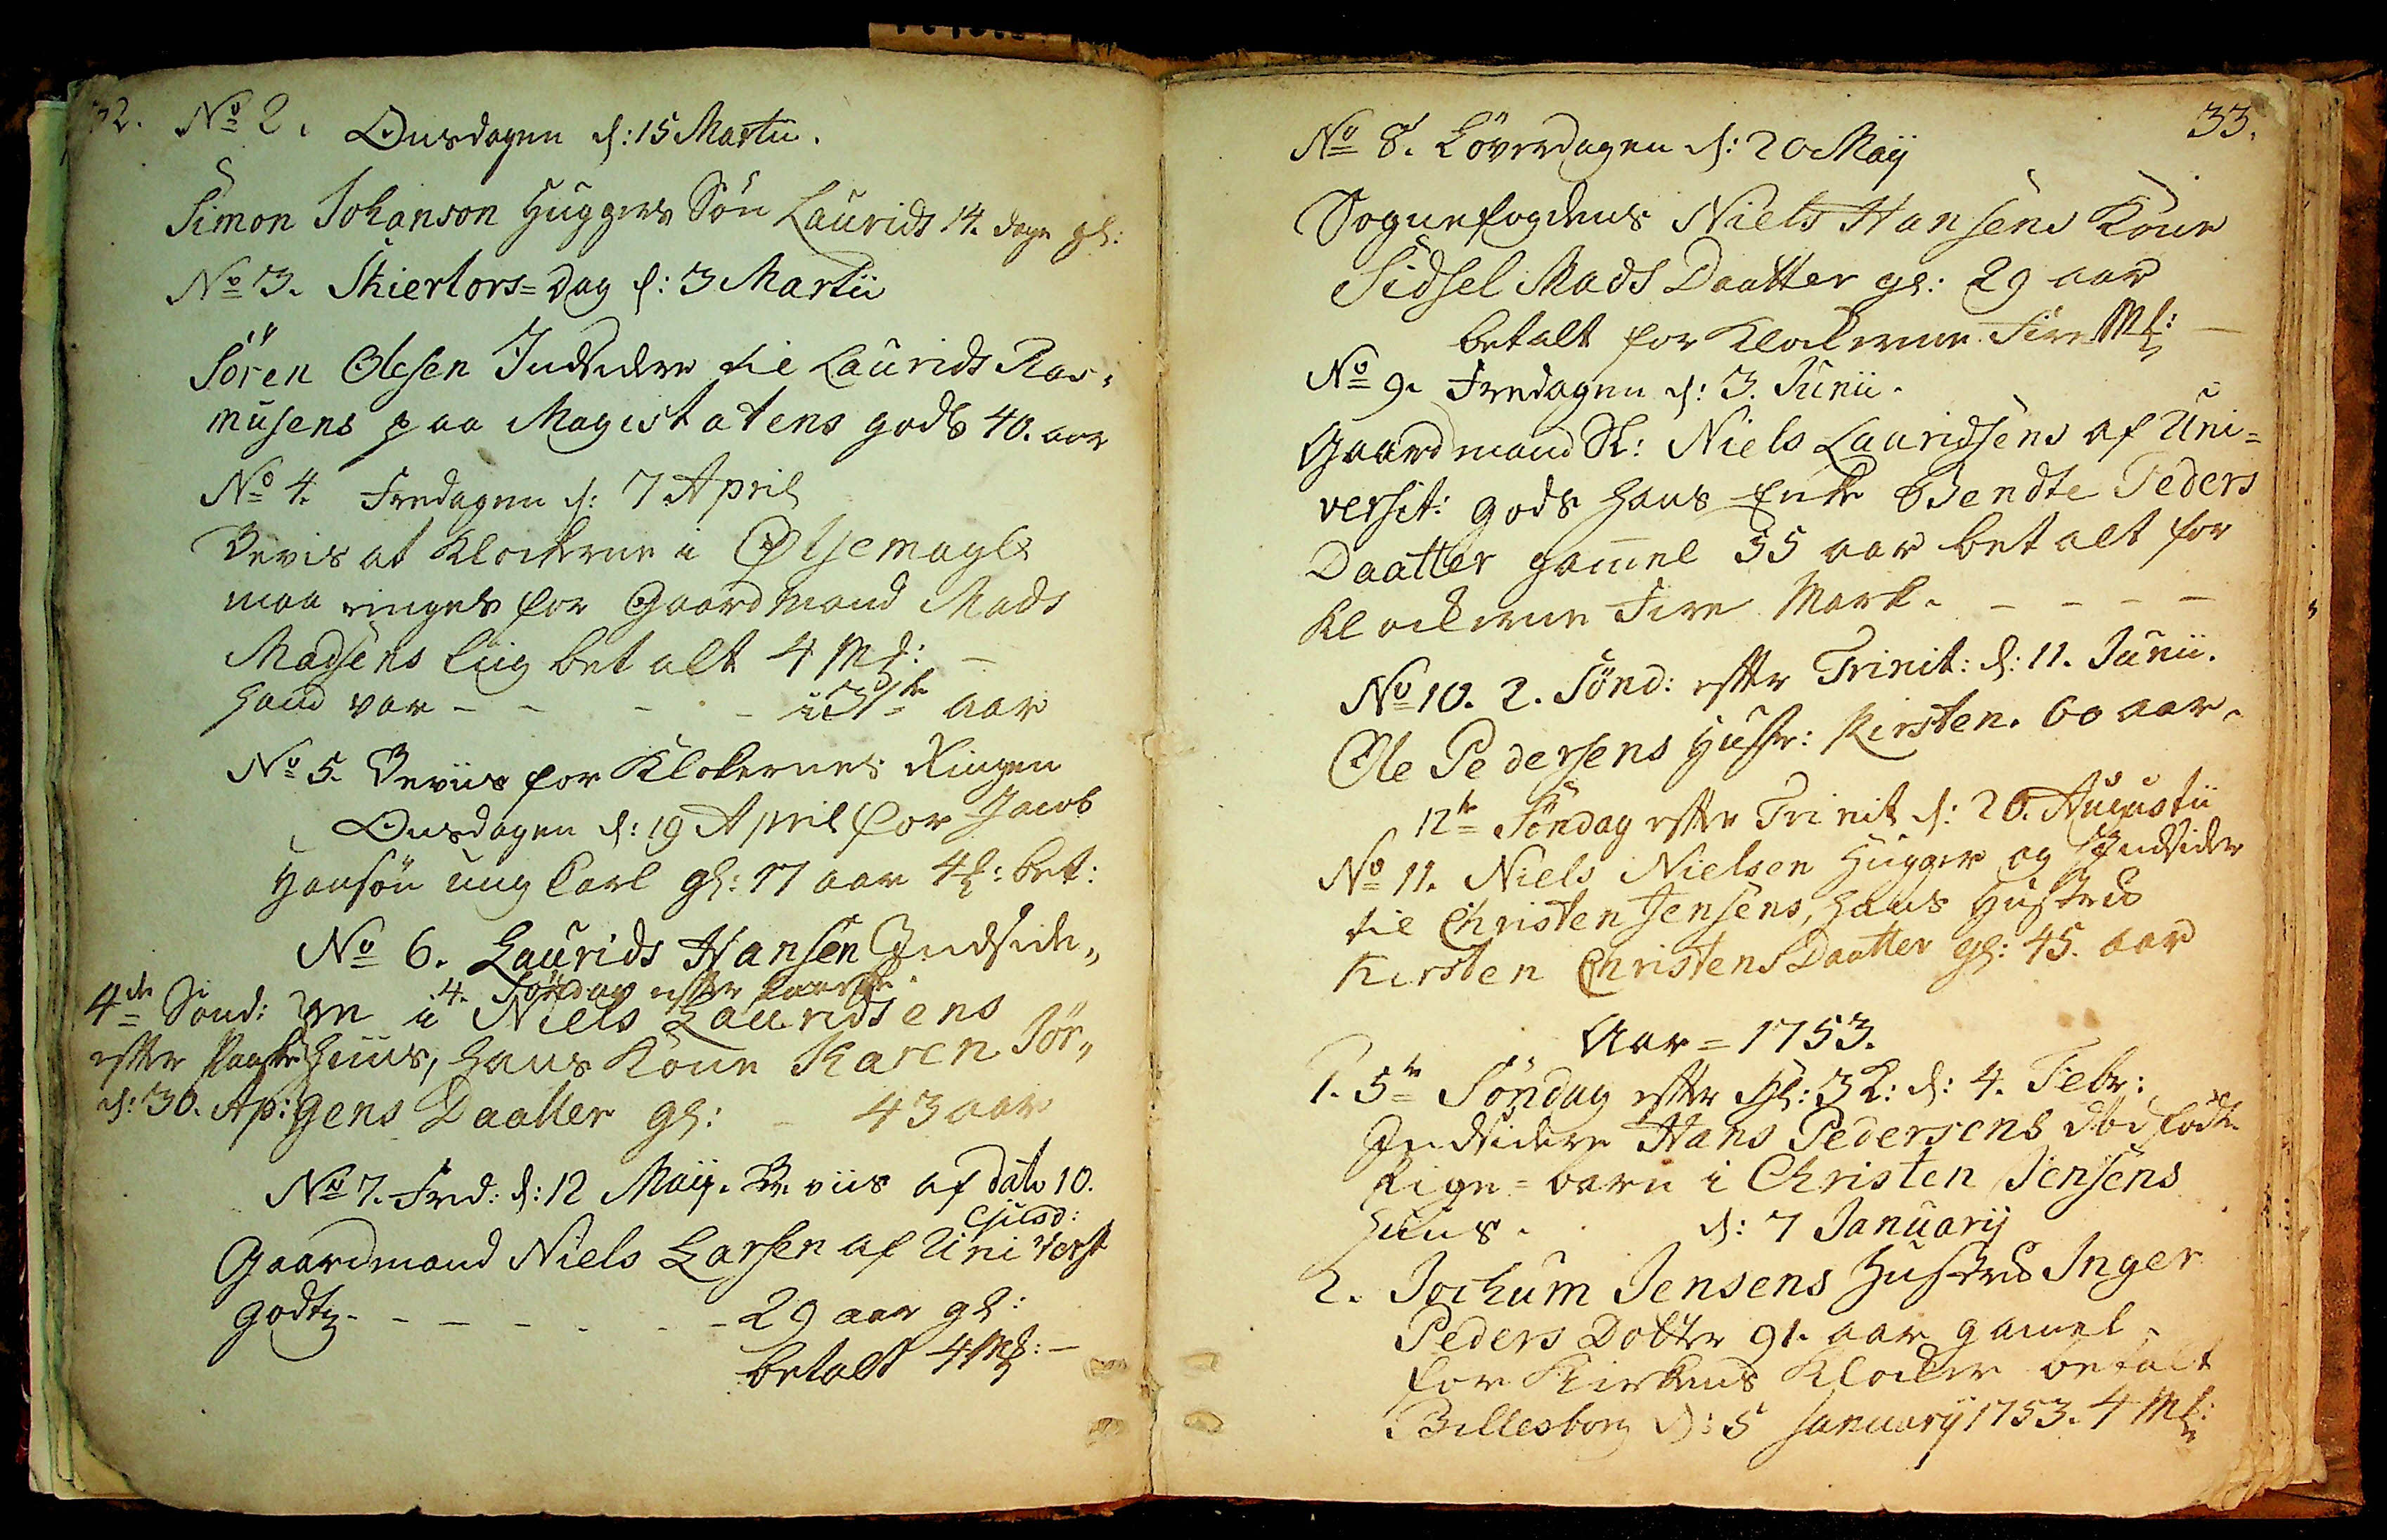32.
No 2. Onsdagen d: 15 Martii.
Simon Johanson Huggers Søn Laurids 14. dage gl:
No 3. Skiertors=Dag d: 3 Martii
Søren Olesen Indsider til Laurids Ras¬
musens paa Magistratens gods 40. aar
No 4. Fredagen d: 7 April
Bevis at Klockerne i Ølsemagle
maa ringes for Gaardmand Mads
Madsens Liig betalt 4 Mk:
Hand var
i 31te aar
No 5. Beviis for Klockernes Ringen
Onsdagen d: 19 April for Jacob
Hansøn Ung karl gl: 17 aar 4m: bet:
No 6. Laurids Hansen Jndsid¬
4. Søndag efter Paaske.
4de Sønd: er i Niels Lauridsens
efter Paaske Huus, hans Kone Karen Jør¬
d: 30.Ap: gens Daatter gl: - 43 aar
No 7. Fred: d: 12 Maij. Beviis af Dato 10.
Ejusd:
Gaardmand Niels Larsen af Universt
Godtz
29 aar gl:
betalt 4 Mk:
33.
No 8. Løverdagen d: 20 Maij
Sognefogedens Niels Hansens Kone
Sidsel Mads Daatter gl: 29 aar
betalt for Klockerne Fire Mk:
No 9. Fredagen d: 3. Junii.
Gaardmand Sl: Niels Lauridsens af Uni¬
versit: Gods hans Enke Bendte Peders
Daatter gammel 55 aar betalt for
Klockerne Fire Mark
No 10. 2. Sønd: efter Trinit: d: 11. Junii.
Ole Pedersens Hustr: Kirsten. 60 aar.
12te Søndag efter Trinit d: 20. Augustii
No 11. Niels Nielsen Hugger og Indsider
til Christen Jensens, hans Hustru
Kirsten Christens Daatter gl: 45. aar
Aar = 1753.
1. 5te Søndag efter Hl: 3 K: d: 4. Febr:
Indsider Hans Pedersens dødfødte
Pige=barn i Christen Jensens
Huus.
d: 7 Januarij
2. Jochum Jensens Hustru Inger
Peders Dotter 91. aar gammel.
for Kirkens Klocker betalt
Billesborg d: 5 Januarij 1753. 4 Mk:
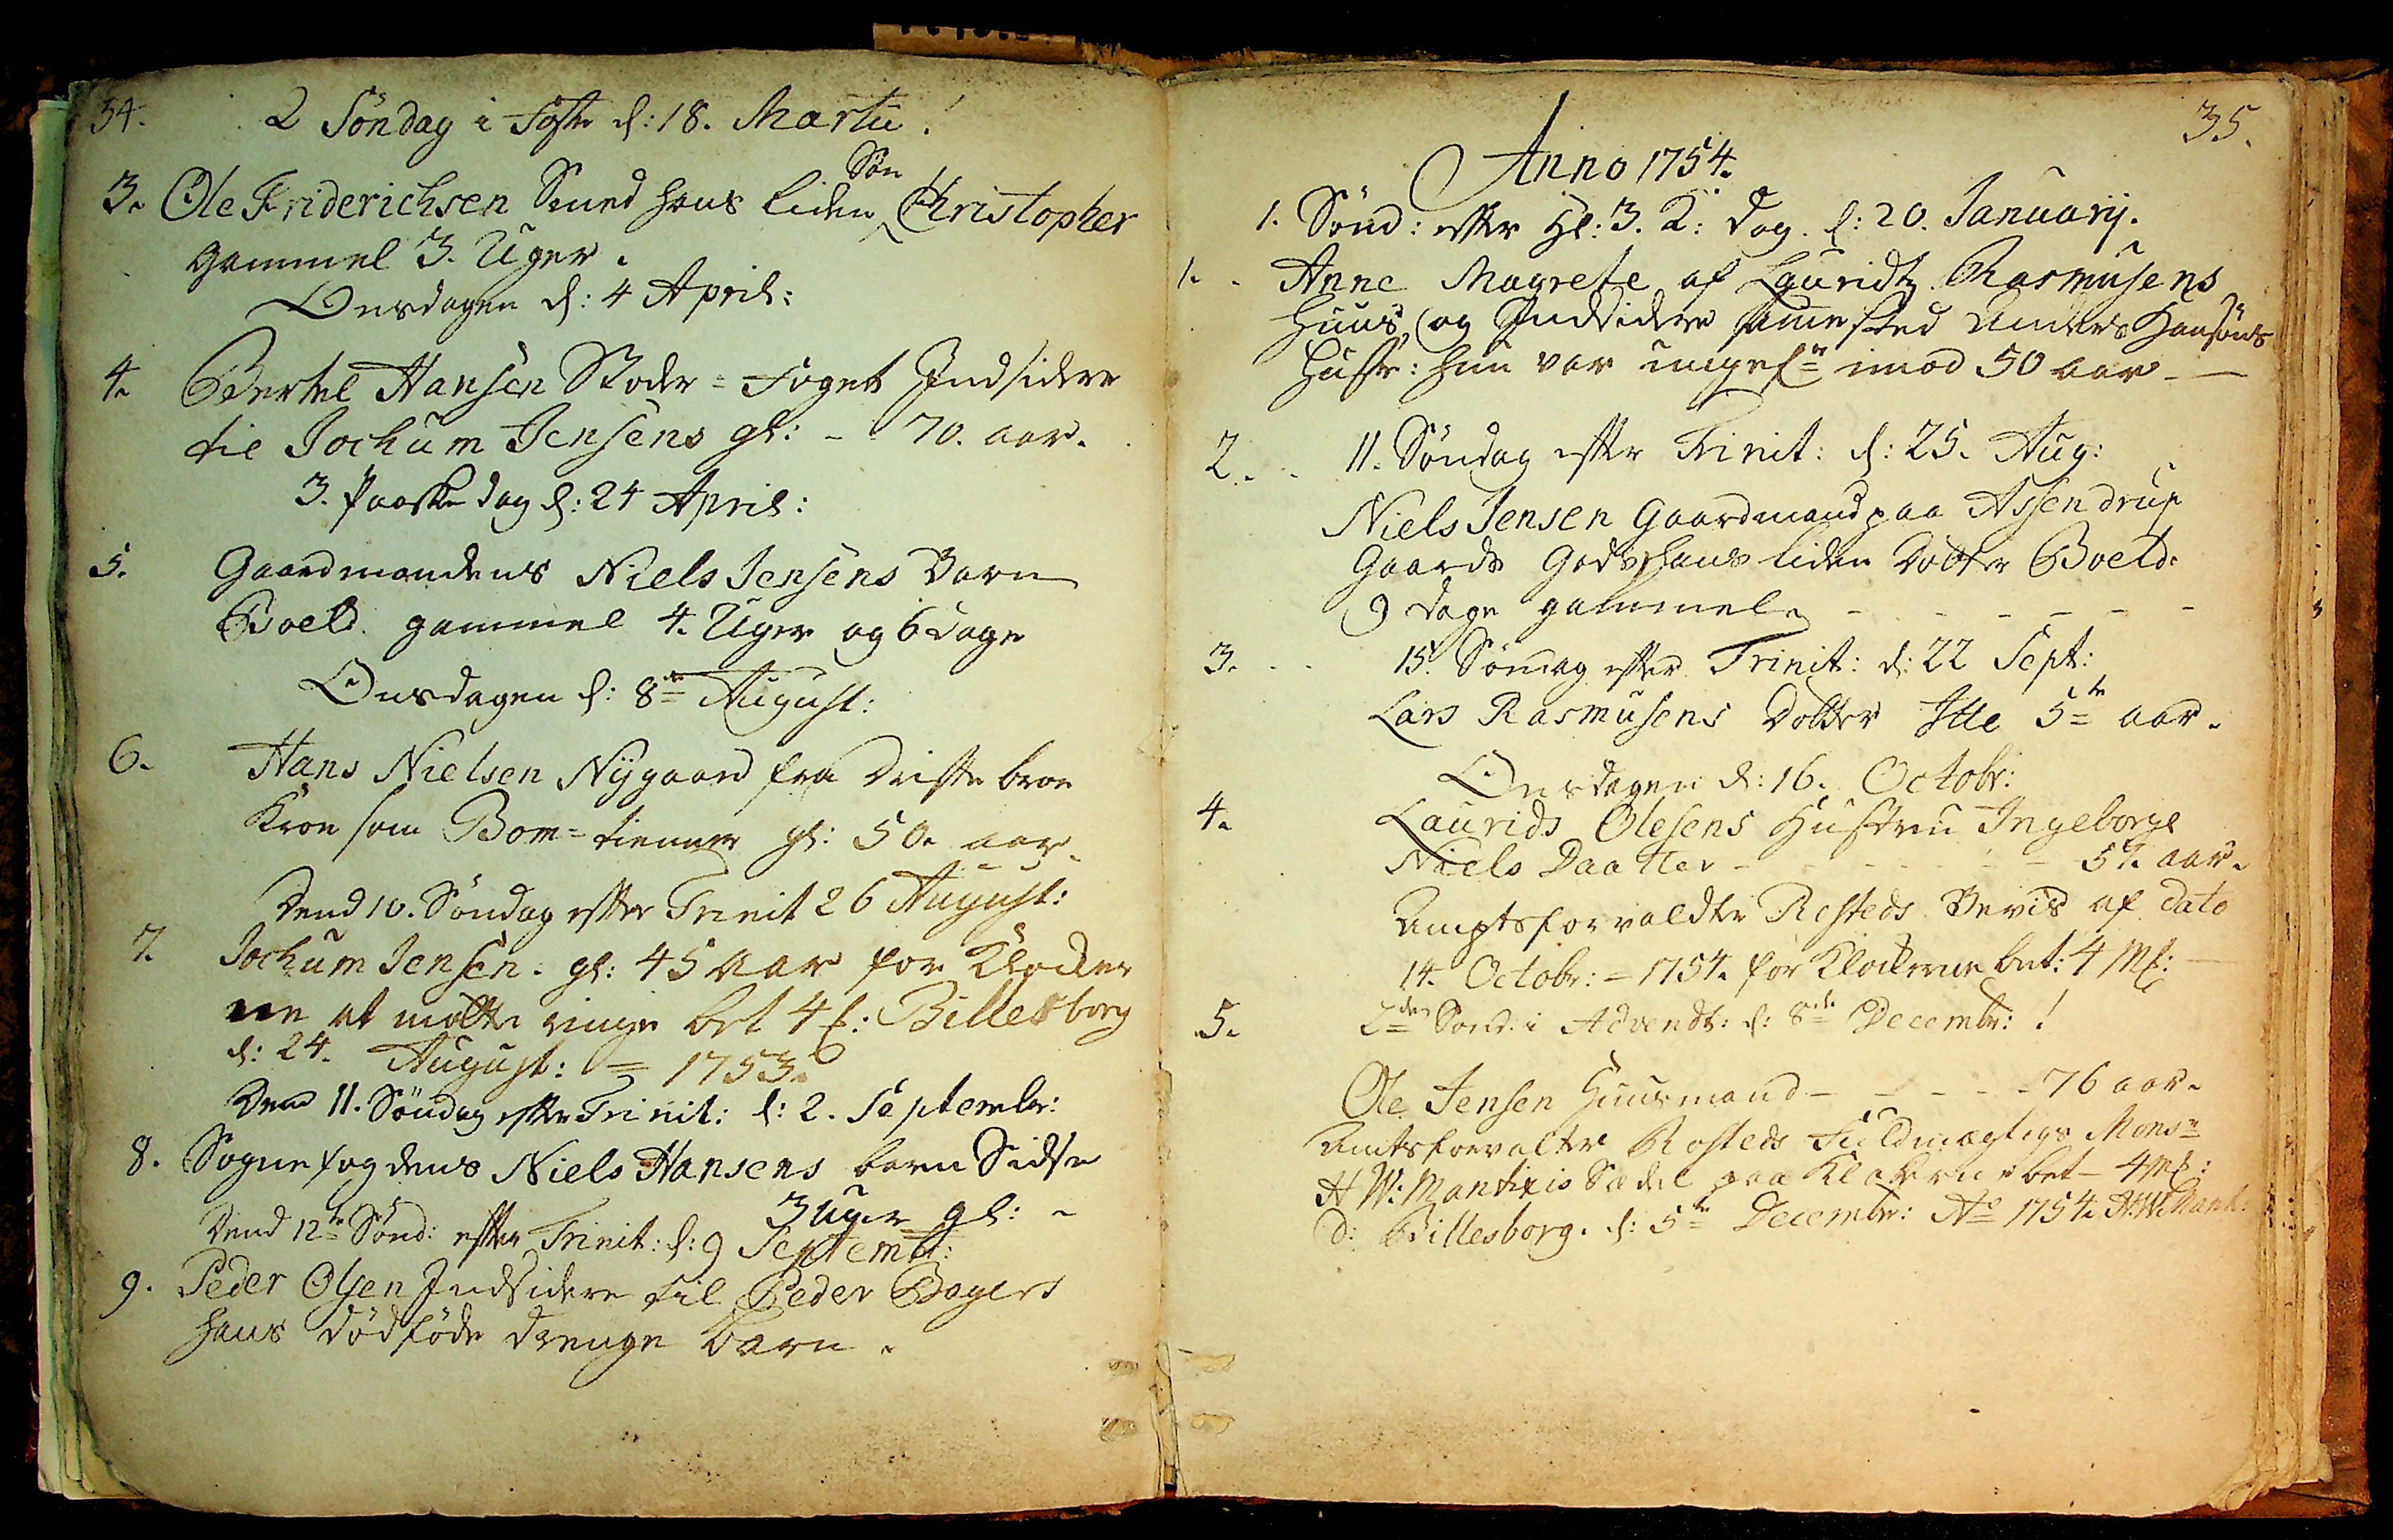34.
2 Søndag i Faste d: 18 Martii.
Søn
3. Ole Friderichsen Smed hans liden Christopher
Gammel 3. Uger.
Onsdagen d: 4 April:
4. Bertel Hansen Stoder=Foget Indsider
til Jochum Jensens gl: - 70. aar.
3. Paaskedag d. 24 April
5. Gaardmandens Niels Jensens Barn
Boeld. gammel 4. Uger og 6 Dage
Onsdagen d: 8de August:
6. Hans Nielsen Nygaard fra Driftebroe
Kroe som Bom=tiener gl: 50. aar.
Dend 10. Søndag efter Trinit 26 August:
7. Jochum Jensen. gl: 45 Aar for Klocker¬
ne at motte ringe bet 4 M: Billesborg
d: 24. August: = 1753.
Dend 11. Søndag efter Trinit: d: 2. Septembr:
8. Sognefogdens Niels Hansens barn Sidse
3 Uger gl: -
Dend 12te Sønd: efter Trinit: d: 9 Septembr:
9. Peder Olsen Indsidere til Peder Bagers
hans dødføde drenge barn.
35.
Anno 1754.
1. Sønd: efter Hl: 3. K: dag. d: 20. Januarij.
1 . . Anne Magrete af Lauridtz Rasmusens
Huus og Indsidere sammested Anders Hansens
Hustr: hun var ungefr imod 50 Aar.
2. . 11. Søndag efter Trinit: d: 25. Aug:
Niels Jensen Gaardmand paa Assendrup
Gaards Gods, hans liden Daater Boeld.
9 Dage gammel
3. . . 15. Søndag efter Trinit: d: 22 Sept:
Lars Rasmusens Dotter Jtte## 5te Aar.
Onsdagen d: 16. Octobr:
4. Laurids Olesens Hustru Ingeborge
Niels Daatter  
57. Aar.
Amptsforvaldter Rosteds Bevis af dato
14. Octobr:=1754: for Klockerne bet: 4 Mk: -
5. 2den Sønd: i Advendt: d: 8de Decembr: 
Ole Jensen Huusmand 
76 Aar.
Amtsforvalter Rosteds Fuldmægtigs Monsr
H W:Mantixis Sædel paa Klockerne bet - 4 Mk:
d: Billesborg. d: 5te Decembr: Ao 1754 H:W. Mant: